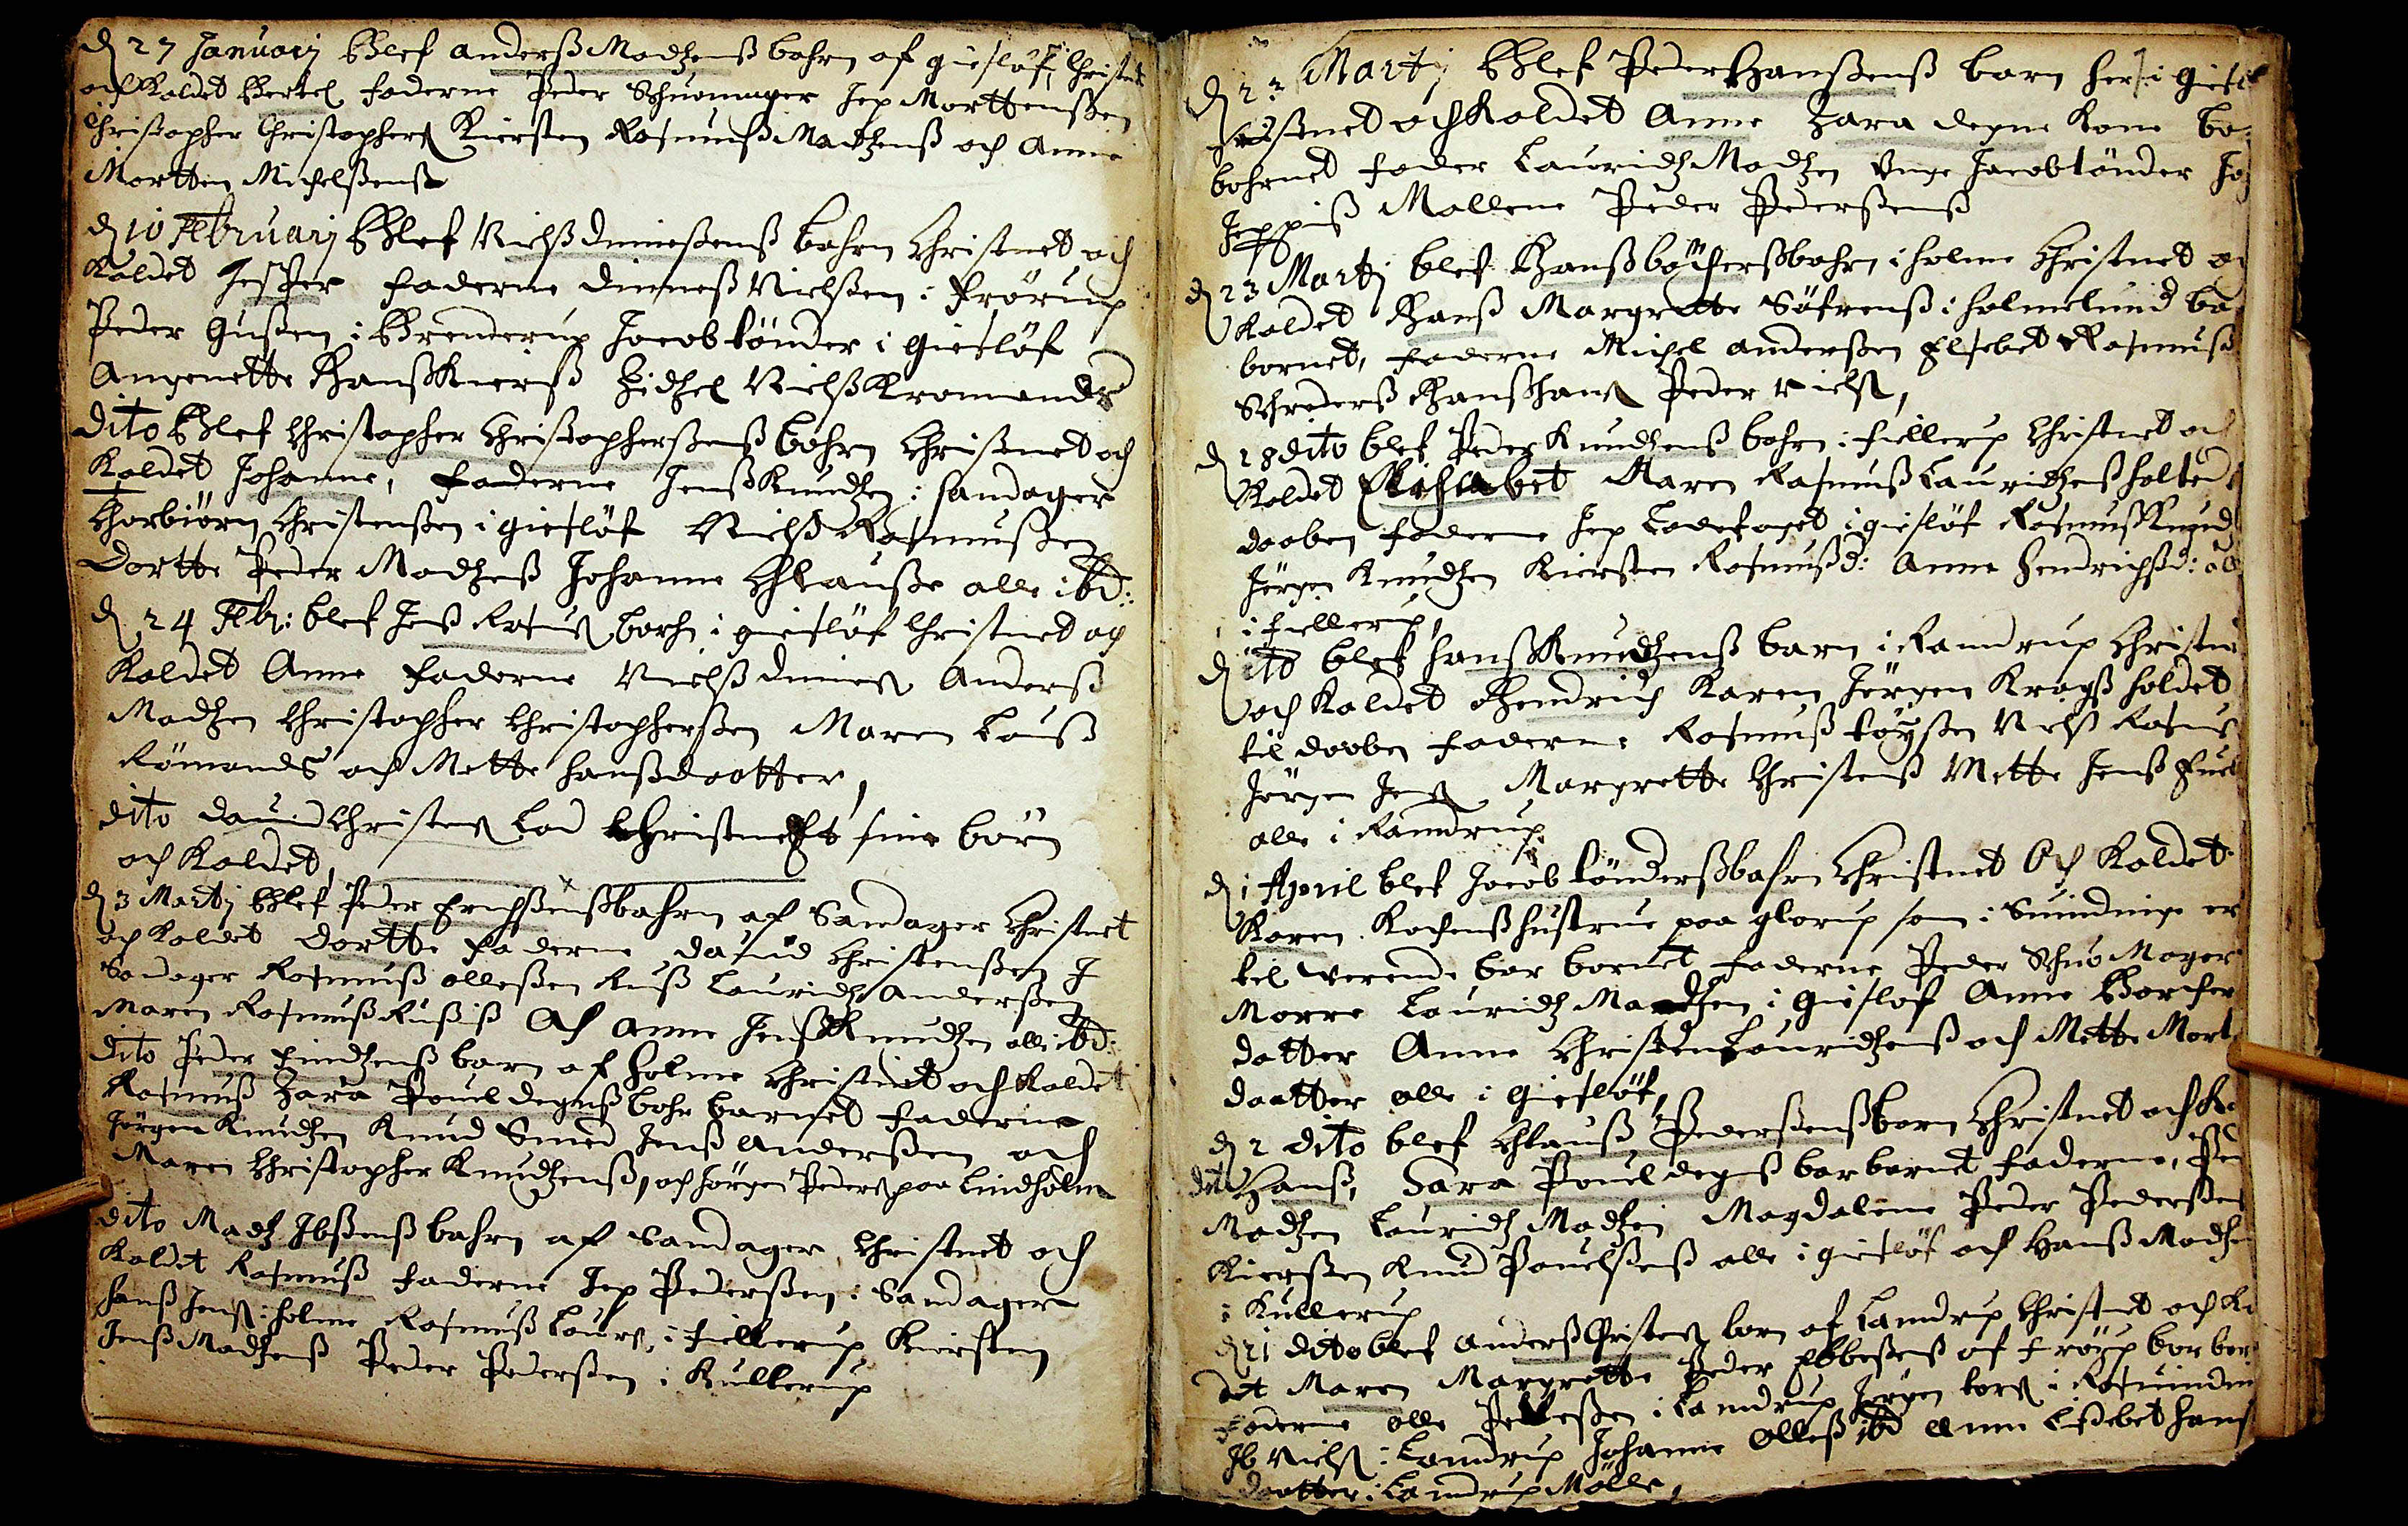d 27 Januarij Blef Anderss Madtzenss bahrn af Gisløf christen
och Kolds Bertel Faderen Peder Schuomager Jep Morttenssen
Christopher Christophers Kiersten Rasmuss Madtzens och Anne
Mortten Michelssenss
d. 10 Februarij Blef Nielss Dinnessenss bahrn Christnet och
kaldet Jesper Faderene Dinness Nielsen i Frørup
Peder Gussen i Brenderup Jacob tønder i Giesløf
Angenette Hanss Kierss Zidtzel Nielss Kromands
dito Blef Christopher Christopherssenss bahrn Christnet och
kaldet Johanne, Faderne Jenss Knudtzen i Sandager
Thorbiørn Christenssen i Giesløf Niels Rasmussen
Dortte Peder Madtzenss Johanne Chlausse alle ibd:.
d 24 Febr: blef Jenss Rasmuss barhn i giesløf christnet och
kaldet Anne Faderne Nielss Dinness Anderss
Madtzen Christopher Christopherssen Maren Lauss
Rømands och Mette Hanssdaatter,
dito Dauid Christens lod christenet sine børn
och kaldet
d 3 Martij Blef Peder Er##chssenss bafrn af Sandager Christnet
och kaldet Dortte faderne Dauid Christensen I
Sandager Rasmuss Ollessen Russ Lauridtz Anderssen
Maren Rasmuss Russiss och Anne Jens Knudtzen alle ibd:.
dito Peder Findtzenss barn af Holme Christnet och kaldet
Rasmuss Zara Pouel Degnss bahr barnet Faderne
Jørgen Knudtzen Knud Smed Jenss Anderssen och
Maren Christopher Knudtzenss, och Jørgen Peders paa Lindholm
dito Madtz Ibssenss bahrn af Sandagen, christnet och
kaldet Rasmuss Faderne Jep Pedersen i Sandager
Hans Jens i Holme Rasmuss Laurs i Fiellerup Kiersten
Jens Madtzens Peder Pederssen i Kullerup
d 23 Martij Blef Peder Hanssenss barn her i Gies 
cristnet och kaldet Anne Zara degne Kone ba 
bahrnet Fader Lauridtz Madtzen vnge Jacob tønder
Jeppiss Mallene Peder Pederssenss
d 23 Martij bleff Hanss Bøcherss bahrn i Holme Christnet oc 
kaldet Hanss Margrette Søfrenss i Holmelund ba
barnet, Faderne Michel Anderssen Elsebet Rasmuss
Schrederss Hanss Hans Peder Niels,
d 18 dito blef Peder Knudtzenss bahrn i Fiellerup Christnet och
kaldet Elisabet Maren Rasmuss Lauridtzenss holte
daaben Faderne Jep Ladefoget i giesløf Rasmuss Knudtz
Jørgen Knudtzen Kiersten Rosmussd: Anne Hendrichsd:
i Fiellerup
dito blef Hanss Knudtzens barn i Ramdrup Christne
och kaldet Hendrich Karen Jørgen Krags holdet
til daaben Faderne Rasmuss tøyssen Nielss Rasmus
Jørgen Jens Margrette Christenss Mette Jenss Fuel 
alle i Ramdrup
d 1 April blef Jacob Tønderss bahrn Christnet Och kaldet
Karen Kochenss hustrue paa Glorup som i Suindinge er
til verende bar barnet Faderne Peder Schuo Mager
Morre Lauridtz Mardtzen i Giesløf Anne Borcher
datter Anne Christen Lauridtzenss och Mette Mort
daatter alle i Giesløf,
d 2 dito blef Chlauss Pederssenss barn christnet och ka
det Hanss Sara Pouel degns bar barnet. Faderen Ped
Madtzen Lauridtz Madtzen Magdalene Peder Pederssens
Kirsten Knud Pouel sens alle i Gietløf och Hanss Madsen
i Kullerup
d 21 dito blef Anders Christens barn af Lamdrip Christet och ka
det Maren Margrette Peder Ebbessenss af Frørup bar bar
Faderen Olle Pederssen i Lamdrup Jørgen tors i R##svindin 
Ib Niels i Lamrup Johanne Olless ibd Anne Essebet Hans
daatter i Lamdrup Mølle,
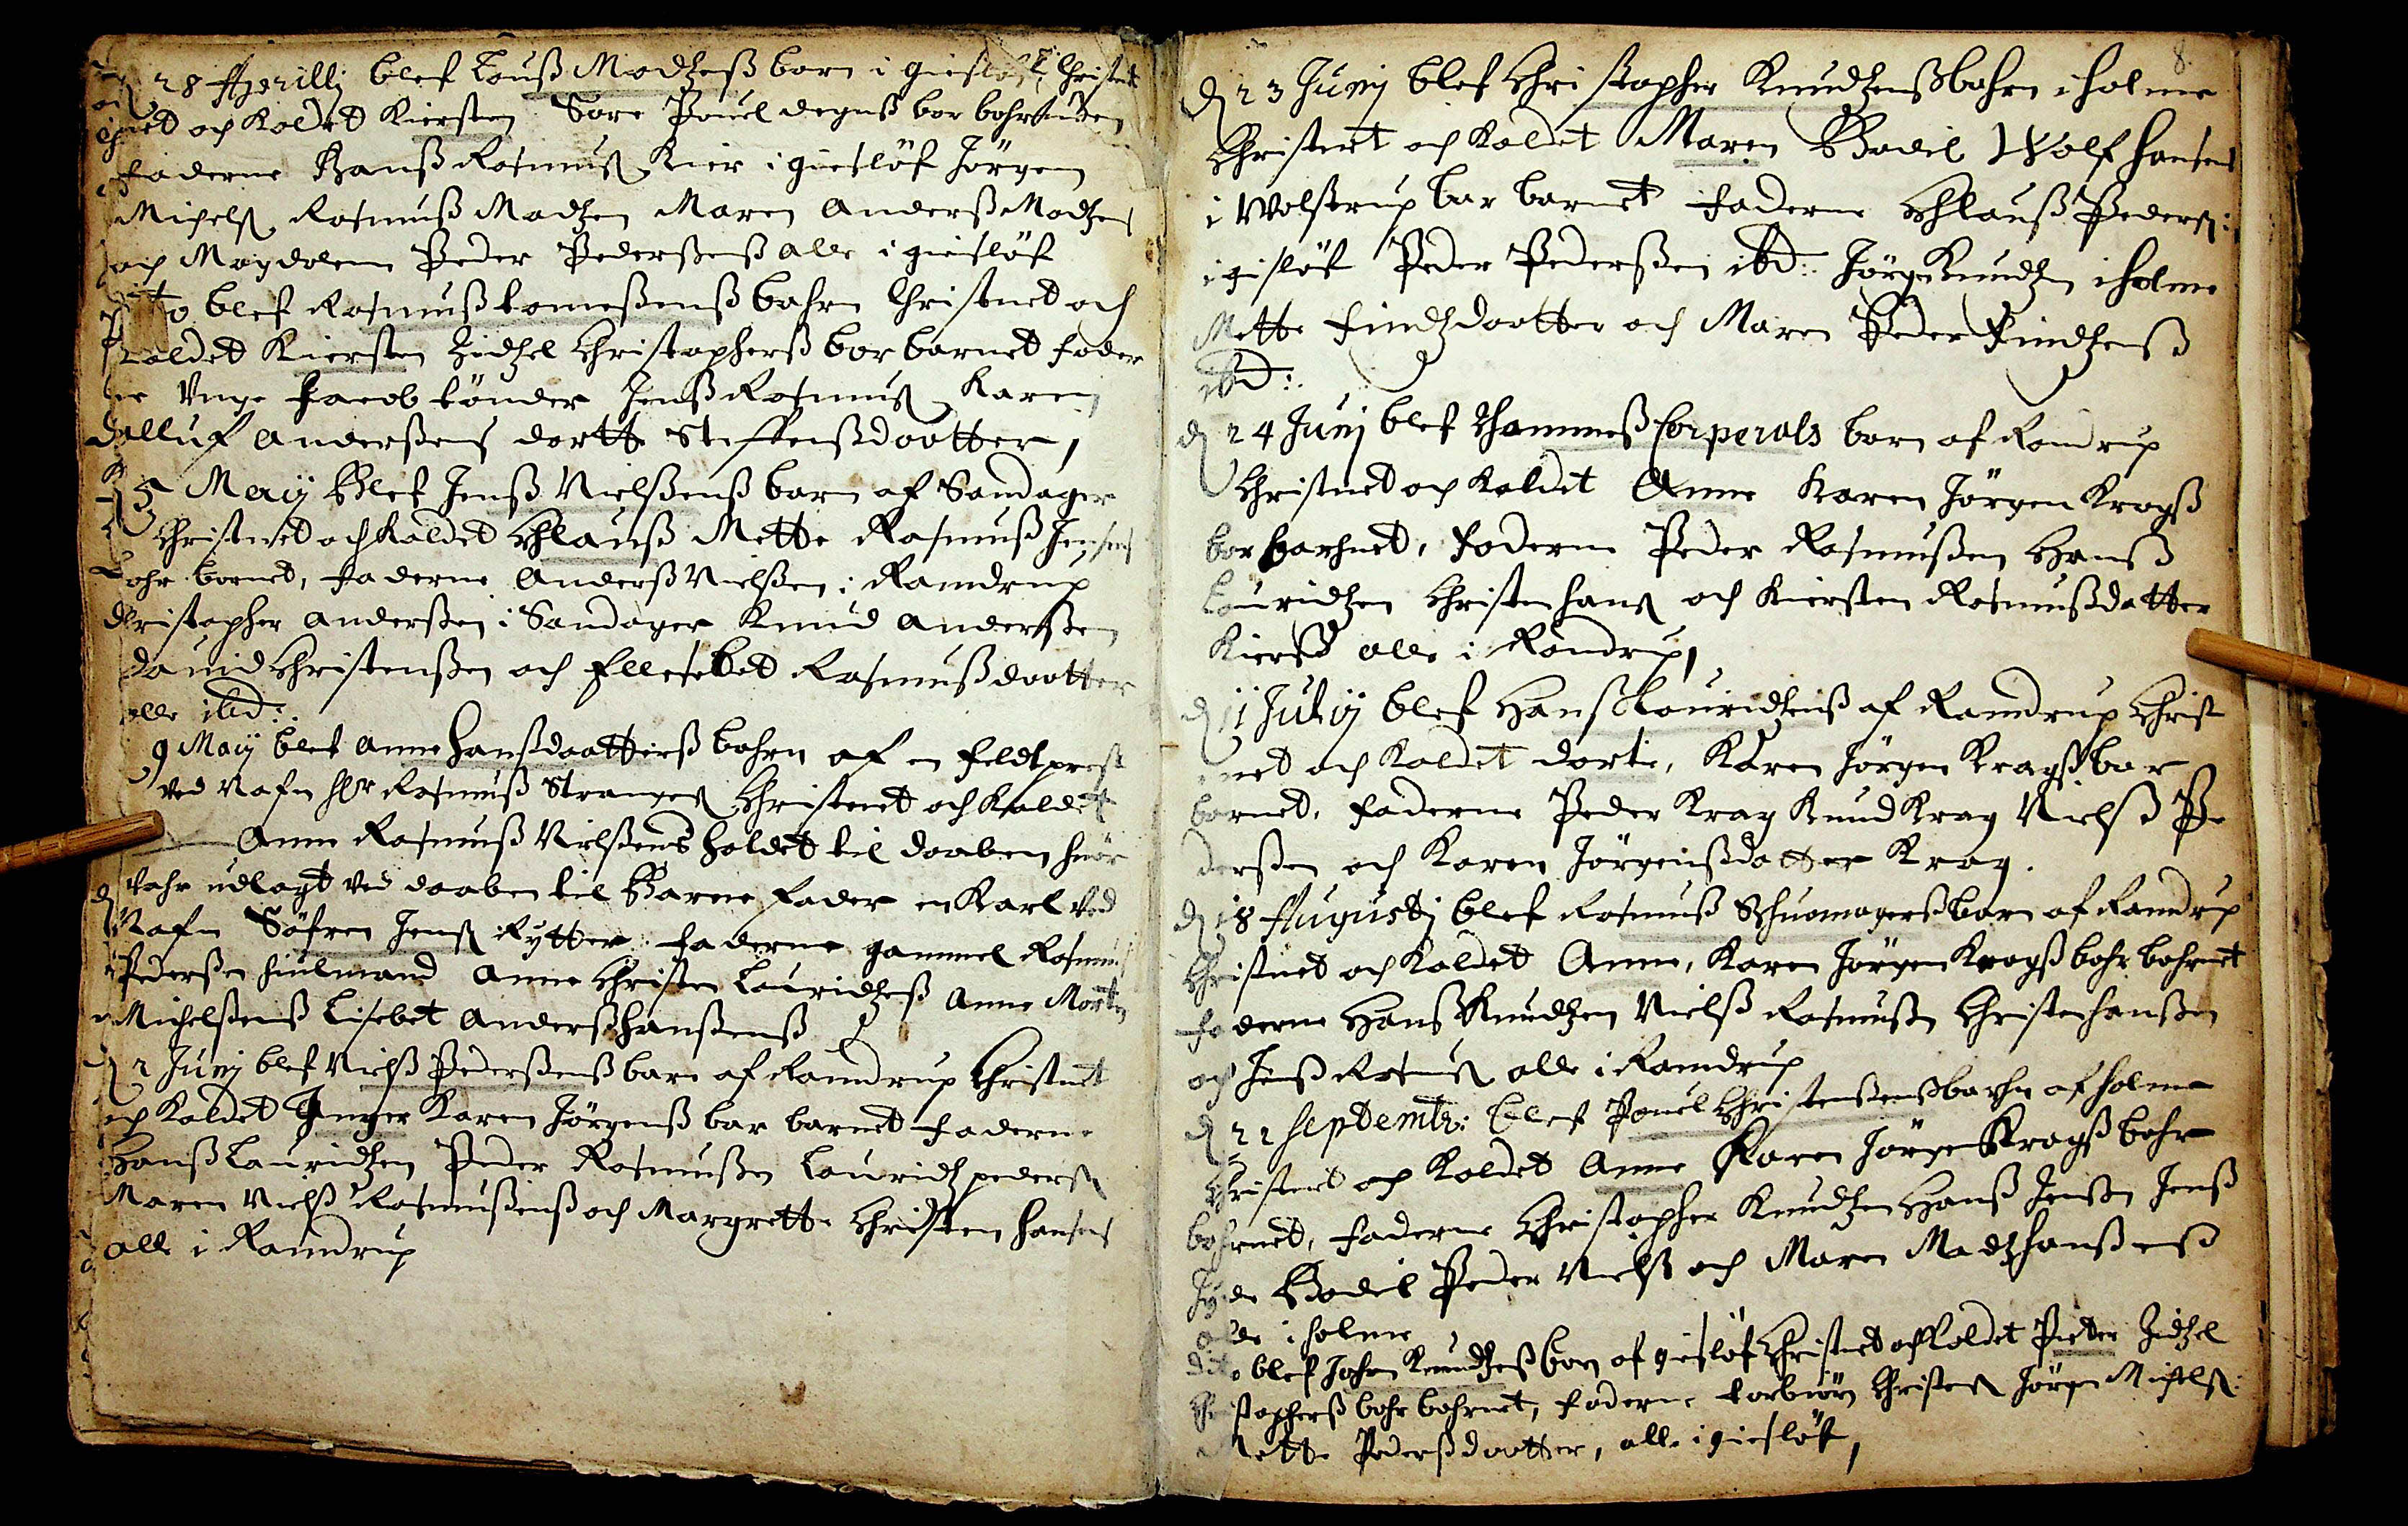d 28 Aprillij blef Lauss Madtzenss barn i Giesløf 
och kaldet Kiersten Sare Pouel degnss bar bahrnet
Faderne Hanss Rosmus Kier i Giesløf Jørgen
Michels Rasmuss Madtzen Maren Anderss Madtzen
och Magdolen Peder Pederssenss alle i giesløf
 blef Rasmuss Tomessenss bahrn Christnet och
kaldet Kiersten Zidtzel Christopherss bar barnet Fader
r vnge Jacob Tønder Jenss Rasmus Karen
Olluf Anderssen Dortte Steffenssdaatter
5. Marij Blef Jenss Nielssenss barn af Sandager
Christenset och kaldet Chlauss Hanss Mette Rasmuss Jensens
ahr barnet, Faderne Anderss Nielssen i Ramdrup
ristopher Anderssen i Sandager Knud Anderssen
Dauid Christenssen och Fllesebet Rasmussdaatter
alle ibd:.
9 Maij blef Anne Hanssdaattirss bahrn af en feldtprest
ved Nafn Her Rofrenss Stranges Christenet och kaldette
__ Anne Rasmuss Nielssens holdet til daaben, h##or
vahr udlagt ved daaben til Barne Fader en Karl ved
Nafn Søfren Jens Rytter. Faderne gammel Rasmus
Pederssen hiulmand Anne Christen Lauridszenss Anne Morten  
Michelssenss Lisebet Anderss Hanssenss
d 1 Junij blef Nielss Pederssenss barn af Ramdrup Christnet
och kaldet Inger Karen Jørgenss bar barnet. Faderne
Hans Lauridtzen Peder Rosmussen Lauridtz pederss
Maren Nielss Rasmussenss och Margrette Christen Hansens
alle i Ramdrup
8.
D 23 Junij blef Christopher Knudtzenss bahrn i Holme
Christnet och kaldet Maren Bodil Wolf Hansens
i Wolstrup bar barnet Faderne Chlauss Peders:
i gisløt Peder Pederssen ibd:. Jørgn Knudtzen i Holme
Mette Findtzdaatter och Maren Peder Findtzenss
ibd:.
d. 24 Junij blef ##hammess Cornperals barn af Ramdrup
Christnet och kaldet Anne Karen Jørgen Kragss
bar barhnet, Faderen Peder Ramussen Hanss
Lauridtzen Christen Hans och Kiersten Rasmussdatter
Kierss alle i Ramdrup,
d 11 Julij blef Hanss Lauridtzenss af Ramdrup Christ
net oc kaldet Dortie, Karen Jørgen Kragss bar
barnet. Faderne Peder Krag Knud Krag Nielss Pe¬
derssen och Karen Jørgenssdatter Krag.
d 18 Augustij blef Rasmuss Schuomagerss barn af Ramdrup
Christnet och kaldet Anne, Karen Jørgen Kragss bahr bahrnet
Faderne Hanss Knudtzen Niels Rasmussen Christen Hanssen
och Jens Rasmus alle i Ramderup
d 22 Sepdembr: blef Pouel Christenssenss barhn af Holme
Christnet och kaldet Anne Karen Jørgen Krags bahr
bahrnet, Faderene Christopher Knudtzen Hanss Jenssen Jenss
Jyde Bodil Peder Nielss och Maren Madtz Hanssens
alle i holme
Dito bleff Johan Kmudtzenss barn af Giesløf Christnet och kaldet Pieter Zidtzel
Christofferss bahr bahrnet, Faderne torbiørn Christens Jørgen Michels
Mette Pederssdaatter, alle i Giesløf,